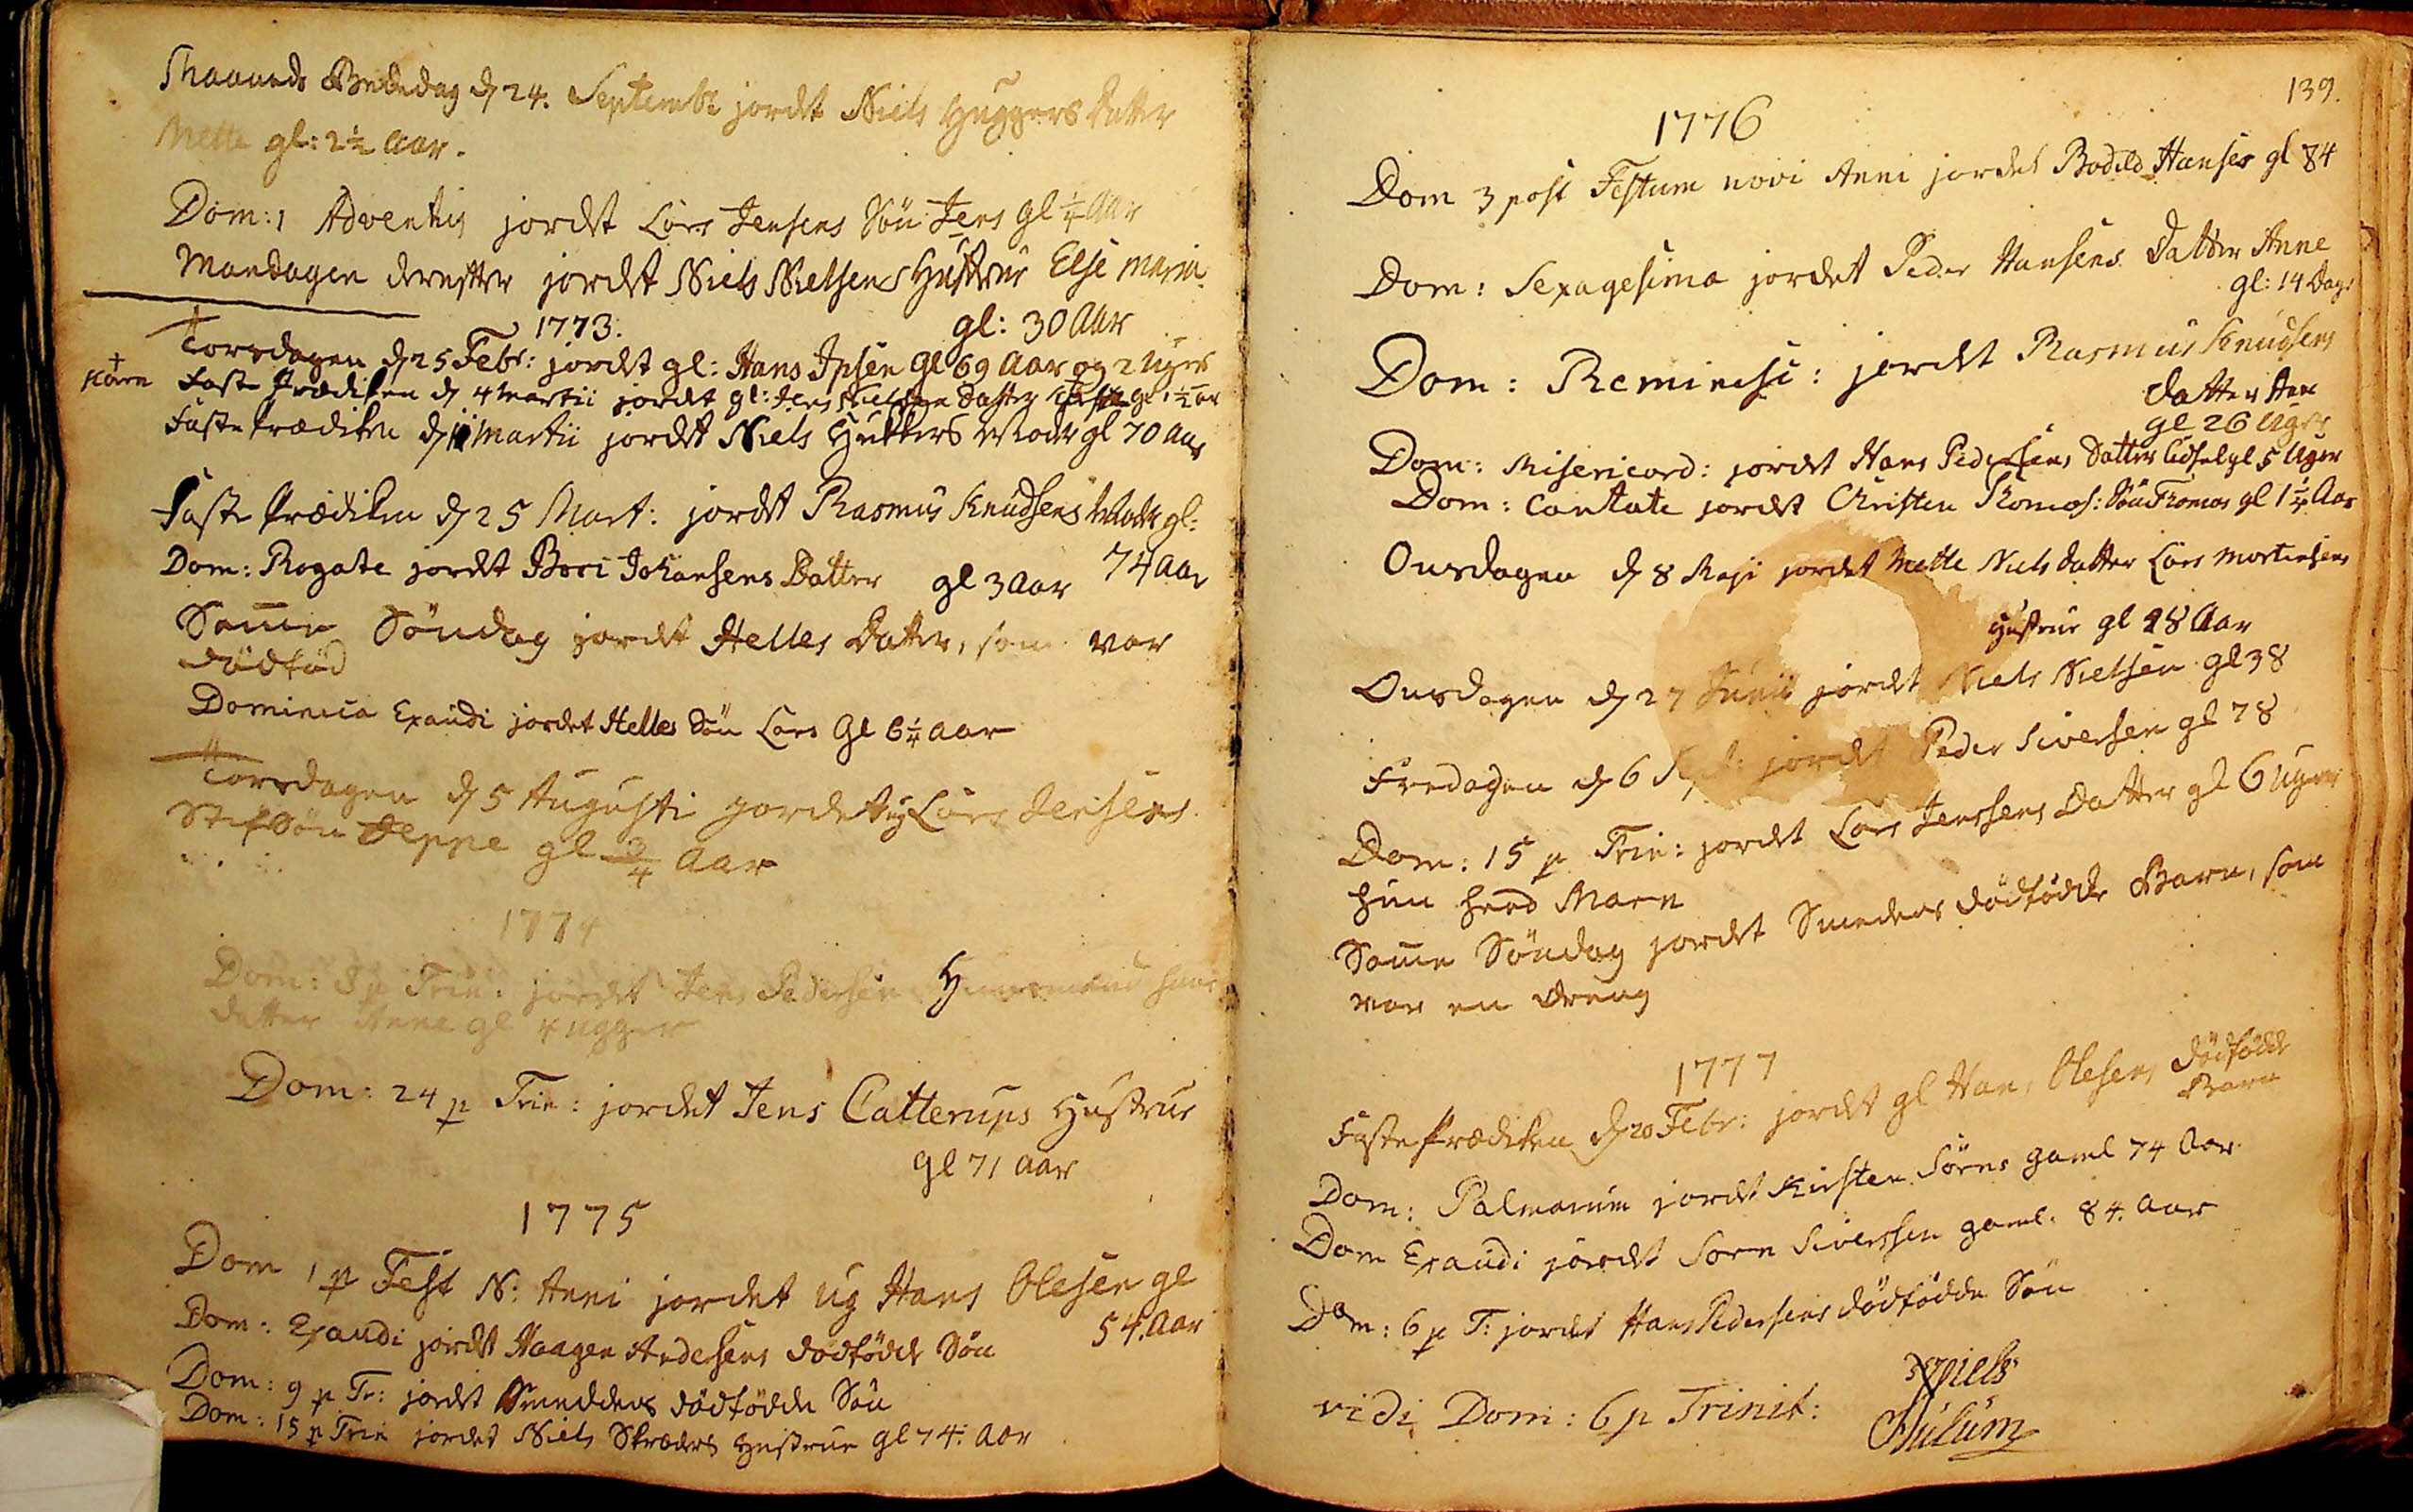Maaneds Bededag d 24. Septembr jordet Niels Huggers Datter
Mette gl: 2½ Aar.
Dom: 1 Adventis jordet Lars Jensens Søn Jens gl ¼ Aar
Mandagen derefter jordet Niels Nielsens Hustrue Else Marie
gl: 30 Aar
1773.
Torsdagen den 5 Febr: jordet gl: Hans Ipsen Gl 69 Aar og 2 Uger
Karn
Faste Prædiken d 4 Martii jordet gl: Jens Nielsens Datter Kirsten gl 1½ ar
Faste Prædiken d 11 Martii jordet Niels Huggers Moder gl 70 Aar
Faste Prædiken d 25 Mart: jordet Rasmus Knudsens Moder gl: 74 Aar
Dom: Rogate jordet Borc Johansens Datter gl. 3 Aar
Samme Søndag jordet Helles Datter, som var
dødfød
Domineca Exaudi jordet Helles Søn Lars Gl 6 ¼ Aar
Torsdagen d 5 Augusti jordet ug Lars Jensens
StefSøn Jeppe gl ¾ Aar
1774.
Dom: 8 p Trin: jordet Jens Pedersen Huusmand hans
Datter Anne gl 4 Ugger
Dom: 24 p Trin: jordet Jens Catterups Hustrue
gl 71 Aar
1775
Dom 1 p Fest N: Anni jordet Ug Hans Olsen gl
54. Aar
Dom: Exaudi jordet Haagen Andersens dødfødde Søn
Dom: 9 p Tr: jordet Smeddens dødfødde Søn
Dom: 15 p Trin jordet Niels Skræders Hustrue gl 74. Aar
139.
1776
Dom 3 post Festum novi Anni jordet Bodild Hanses gl 84
Dom: Sexagesima jordet Peder Hansens Datter Anne
gl: 14 Dage
Dom: Reminesc: jordet Rasmus Knudsens
datter Ane
gl 26 Uger
Dom: Misericord: jordet Hans Pedersens Datter Cidsel gl 5 Uger
Dom: Cantate jordet Christen Thomæs: Søn Thomas gl 1 ¼ Aar
Onsdagen d 8 Maji jordet Mette Niels datter Lars Mortensens
Hustrue gl 28 Aar
Onsdagen d 27 Junii jordet Niels Nielsen gl 38
Fredagen d 6 Sept: jordet Peder Siversen gl 78
Dom: 15 p Trin: jordet Lars Jenssens Datter gl 6 Uger
hun hed Marn
Samme Søndag jordet Smedens dødfødde Barn, som
var en Dreng
1777
Faste Prædiken d 20 Febr: jordet gl Hans Olesens
dødfødde 
Barn
Dom: Palmarum jordet Kirsten Sørns gaml 74 Aar
Dom: Exaudi jordet Sørn Siverssen gaml: 84. Aar
Dom: 6 p T: jordet Hans Pedersens dødfødde Søn
Vidi Dom: 6 p Trinit:
Niels
Aulum 
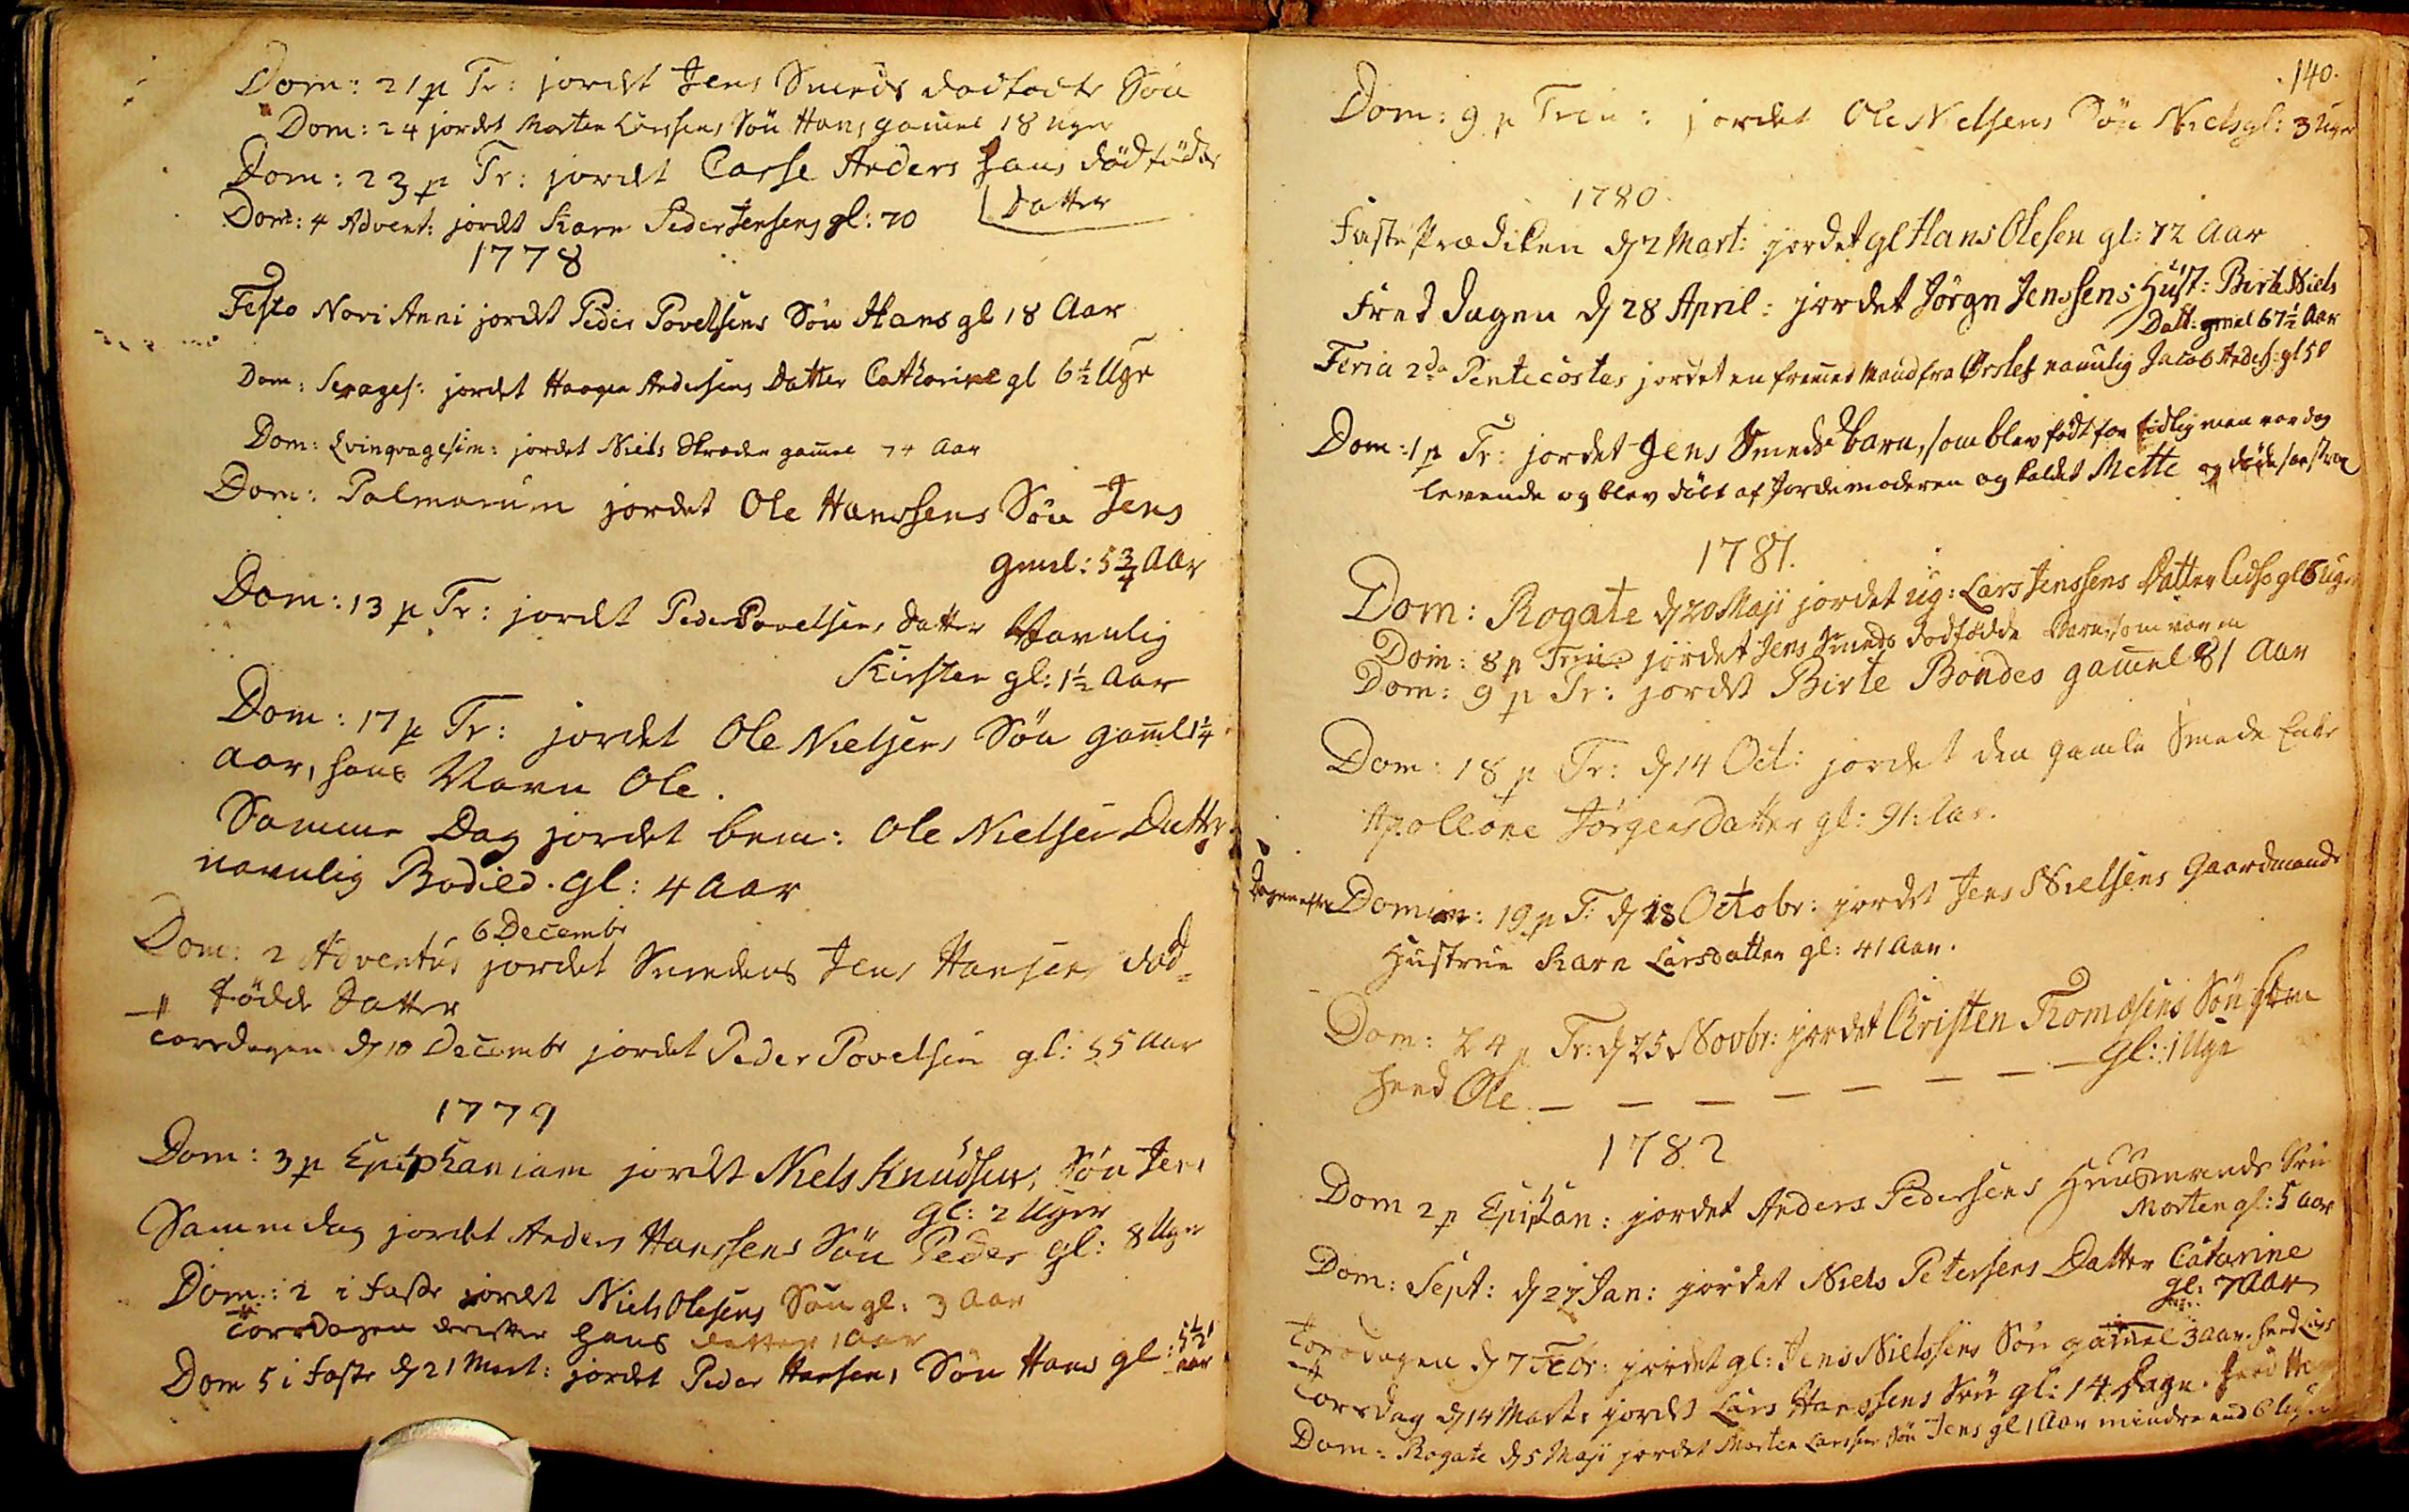Dom: 21 p Tr: jordet Jens Smeds dødfødte Søn
Dom: 24 jordet Morten Larssens Søn Hans gammel 18 Uger
Dom: 23 p Tr: jordet Cashe## Anders hans dødfødde
Datter
Dom: 4 Advent: jordet Karn Peder Jensens gl: 70
1778
Festo Novi Anni jordet Peder Povelsens Søn Hans gl 18 Aar
Dom: Sexages: jordet Haagen Andersens Datter Catharine gl 6½ Uge
Dom: Qvinqvagesim: jordet Niels Skræder gammel 74 Aar
Dom: Palmarum jordet Ole Hanssens Søn Jens
gaml: 5 ¾ Aar
Dom: 13 p Tr: jordet Peder Povelsens datter Navnlig
Kirsten gl: 1½ Aar
Dom: 17 p Tr: jordet Ole Nielsens Søn gamel 1¼
Aar, hans Navn Ole.
Samme Dag jordet bem: Ole Nielsens Datter
navnlig Bodild. gl: 4 Aar
6 Decembr
Dom: 2 Adventus jordet Smedens Jens Hansens død¬
fødde Datter
Torsdagen d 10 Decembr jordet Peder Povelsen gl: 55 Aar
1779
Dom: 3 p Epiphaniam jordet Niels Knudsens Søn Jens
gl: 2 Uger
Sammedag jordet Anders Hanssens Søn Peder gl: 8 Uger
Dom: 2 i Faste jordet Niels Olsens Søn gl: 3 Aar
Torsdagen derefter hans Datter 1 Aar
Dom 5 i Faste d 21 Mart: jordet Peder Hansens Søn Hans gl: 5½
Aar
140.
Dom: 9 p Trin: jordet Ole Nielsens Søn Niels gl: 3 Uger
1780
Faste Prædiken d 2 Mart: jordet gl Hans Olesen gl: 72 Aar
Freddagen d 28 April: jordet Jørgn Jenssens Hust: Birte Niels
Datt: gmel 67½ Aar
Feria 2da Pentecostes jordet en fremed Mand fra Ørslef navnlig Jacob Anders: gl 50
Dom: 1 p Tr: jordet Jens Smeds Barn, som blev født for tidlig men var dog
levende og blev døbt af Jordemoderen og kaldet Mette og døde saa strax
1781.
Dom: Rogate d 20 Maji jordet Ug: Lars Jenssens Datter Cidse gl 6 Uger
Dom: 8 p Trin: jordet Jens Smeds dødfødde Barn som var en
Dom: 9 p Tr: jordet Birte Bondes gammel 81 Aar
Dom: 18 p Tr: d 14 Oct: jordet den gamle Smeds Enke
Apollone Jørgensdatter gl: 91. Aar.
Dagen efterDomin: 19 p T: d 22 Octobr: jordet Jens Nielsens Gaardmands
Hustrue Karn Larsdatter gl: 41 Aar.
Dom: 24 p Tr: 25 Novbr: jordet Christen Thomæsens Søn som
heed Ole
gl: 1 Uge
1782
Dom 2 p Epiphan: jordet Anders Pedersens Huusmands Søn
Morten gl: 5 Aar
Dom: Sept: d 27 Jan: jordet Niels Petersens Datter Catarine
gl: 7 Aar
Torsdagen d 7 Febr: jordet gl: Jens Nielssens Søn gammel 3 Aar. heed Lars
Torsdagen d 14 Mart: jordet Lars Hanssens Søn gl: 14 Dage. heed Ha##
Dom: Rogate d 5 Maji jordet Morten Larssens Søn Jens gl 1 Aar mindre end 6 Uger